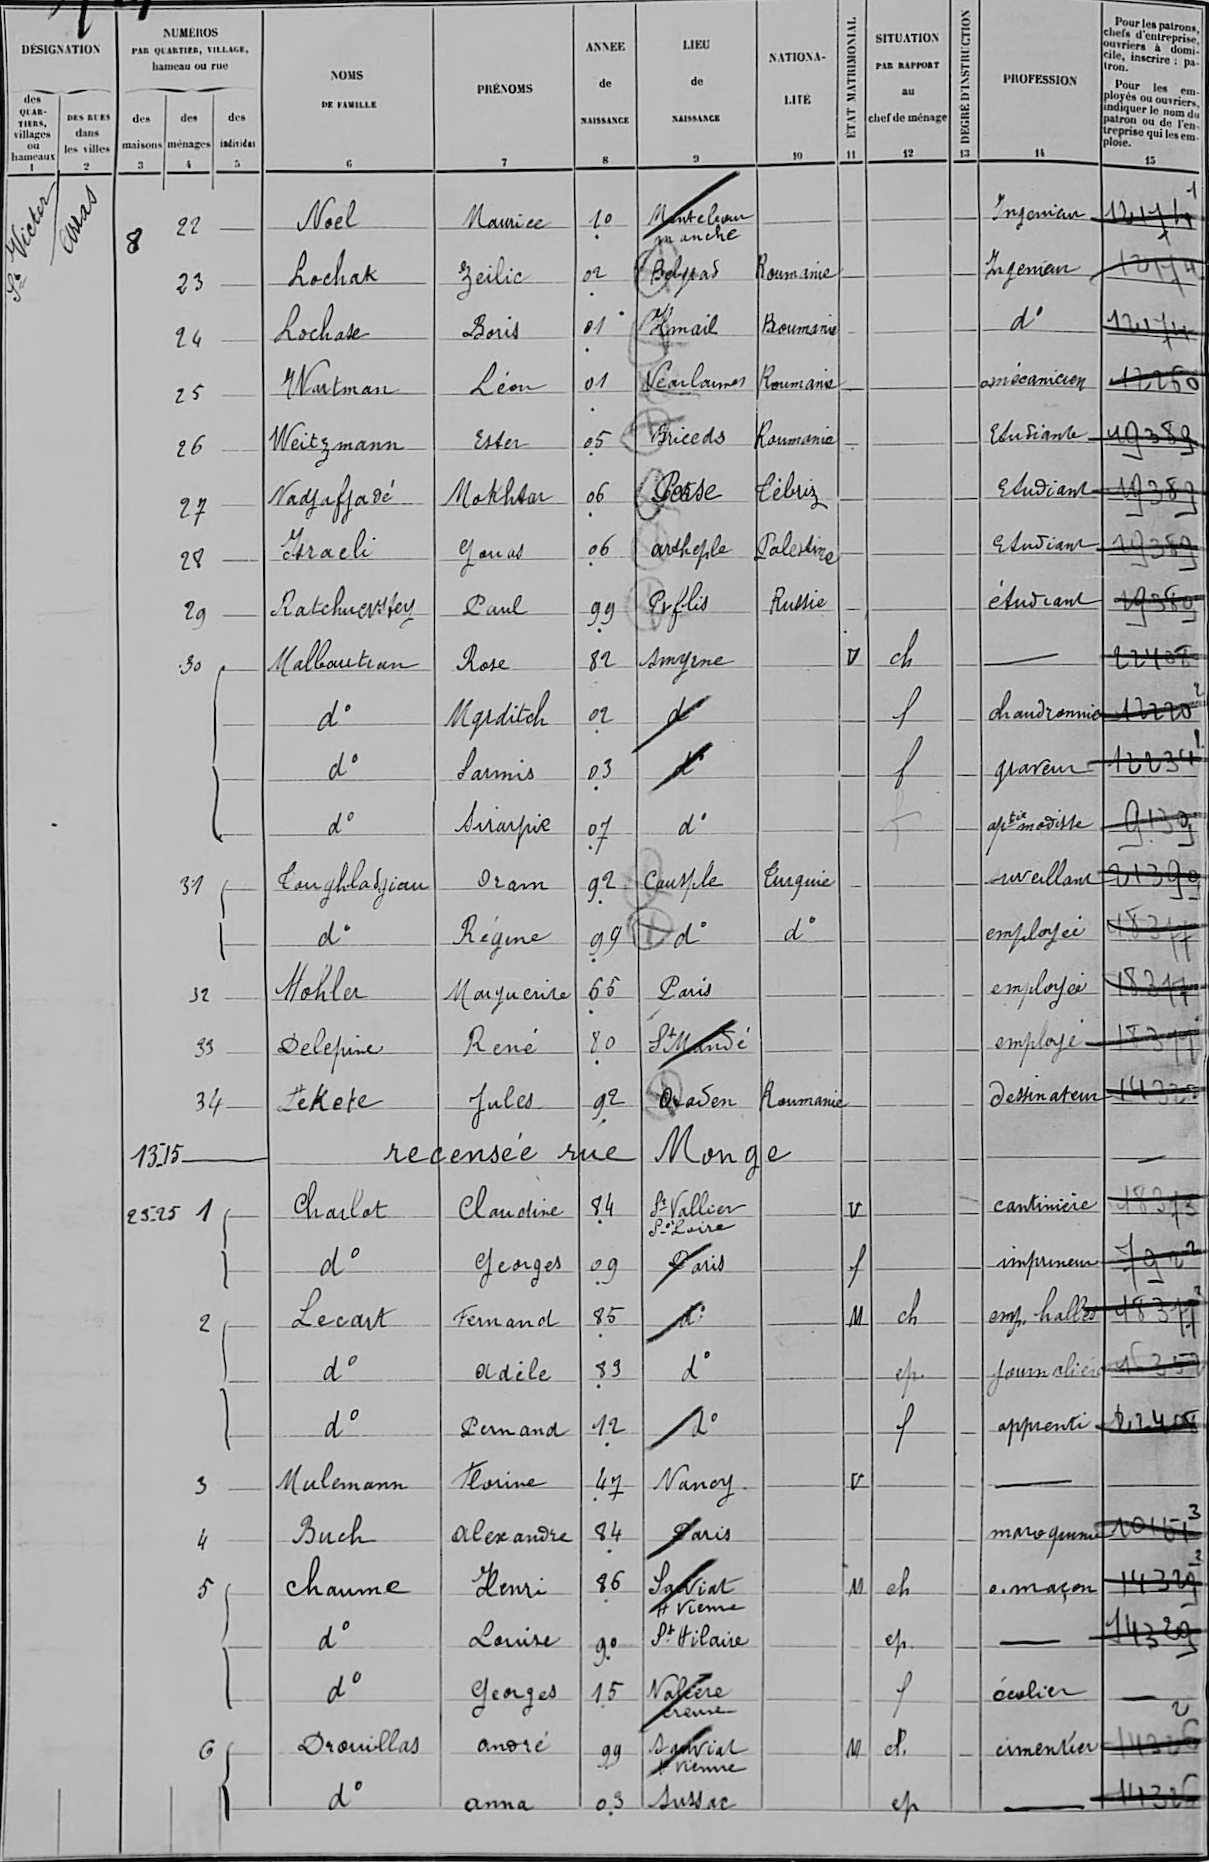Noël/Maurice/10/Monteleour !Manche/¤/¤/¤/¤/Ingénieur/12174
LochaK/Zeilie/02/Belgrad/Roumanie/¤/¤/¤/Ingenieur/12174
Lochase/Boris/01/Kmail/Roumanie/¤/¤/¤/d°/12174
Wutman/Léon/01/Verlaimes/Roumanie/¤/¤/¤/o mécanicien/12260
Weitzmann/Ester/05/Briceds/Roumanie/¤/¤/¤/Etudiante/19389
Nadjafjadé/Makhtar/06/Peae/Tébriz/¤/¤/¤/Etudiant/19389
Israeli/Gonas/06/artheple/Palestine/¤/¤/¤/Etudiant/19389
Ratchuerstey/Paul/99/Pvflis/Russie/¤/¤/¤/étudiant/19389
Malbautran/Rose/82/Smyrne/¤/V/ch/¤/¤/22408
d°/Mgrditch/02/d°/¤/¤/f/¤/chaudronnier/12220
d°/Sarmis/03/d°/¤/¤/f/¤/graveur/12234
d°/Sirarpie/07/d°/¤/¤/f/¤/aptie modiste/9139
Toughladgian/Oram/92/Caspile/Turquie/¤/¤/¤/surveillant/21399
d°/Régine/99/d°/d°/¤/¤/¤/employée/18377
Hohler/Marguerite/66/Paris/¤/¤/¤/¤/employée/18377
Delepine/René/80/St Mandé/¤/¤/¤/¤/employé/18399
Lekete/Jules/2/Ouaden/Roumanie/¤/¤/¤/dessinateur/14328
recensée rue Monge
Charlot/Claudine/84/St Vallier !S & Loire/¤/v/¤/¤/cantinière/18373
d°/Georges/09/Paris/¤/f/¤/¤/imprimeur/792
Lecart/Fernand/85/d°/¤/M/ch/¤/emp halles/18377
d°/Adèle/83/d0/¤/¤/ep /¤/journalière/16352
d°/Pernand/12/d°/¤/¤/f/¤/apprenti/22408
Mulemann/Florine/47/Nancy/¤/v/¤/¤/¤/¤
Buch/Alexandre/84/Paris/¤/¤/¤/¤/maroquinerie/10151
Chaume/Henri/86/Saviat !Hte Vienne/¤/M/ch/¤/o maçon/14329
d°/Louise/90/St Hilaire/¤/¤/ep /¤/¤/14329
d°/Georges/15/Naliere !Creuse/¤/¤/f/¤/écolier/¤
Drouillas/André/99/Sauviat !Hte Vienne/¤/M/ch /¤/cimentier/14326
d°/anna/03/Sussac/¤/¤/ep/¤/¤/14326
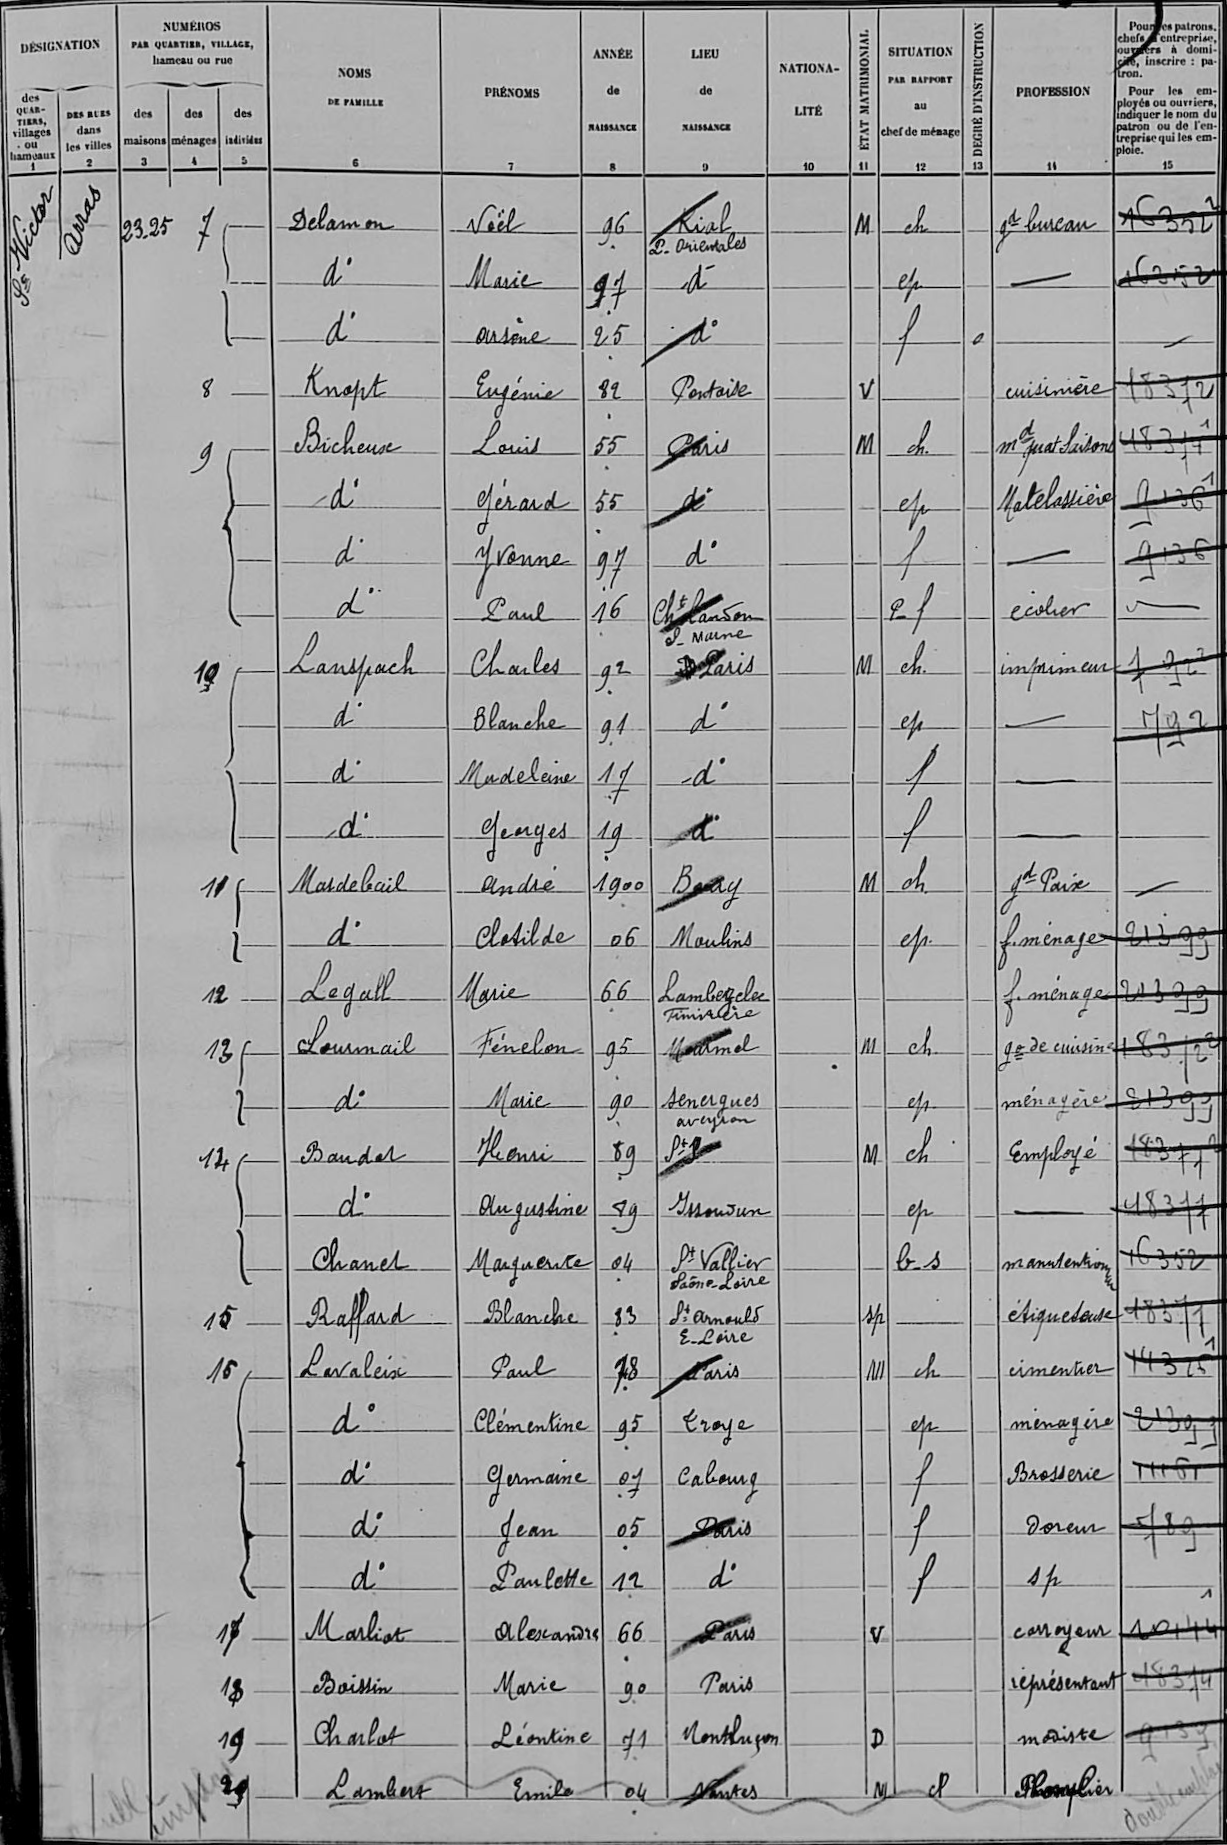Delamon/Noël/96/Rial !P Orientales/¤/M/ch/¤/gd bureau/16352
d°/Marie/97/d°/¤/¤/ep/¤/¤/16352
d°/arsène/25/d°/¤/¤/f/o/¤/¤
Knopt/Eugénie/82/Pontoise/¤/v/¤/¤/cuisinière/18372
Bicheux/Louis/55/Paris/¤/M/ch /¤/md quat Saisons/18374
d°/Gérard/55/d°/¤/¤/ep/¤/Matelassière/9136
d°/Yvonne/97/d°/¤/¤/f/¤/¤/9136
d°/Paul/16/Cht Landon ! S Marne/¤/¤/P f/¤/écolier/¤
Lanspach/Charles/92/Paris/¤/M/ch /¤/imprimeur/792
d°/Blanche/91/d°/¤/¤/ep/¤/¤/792
d°/Madeleine/17/d°/¤/¤/f/¤/¤/¤
d°/Georges/19/d°/¤/¤/f/¤/¤/¤
Masdebail/andré/1900/Bourg/¤/M/ch /¤/gd paix/¤
d°/Clotilde/06/Moulins/¤/¤/ep/¤/f Ménage/21399
Legall/Marie/66/Lambezelec !Finistère/¤/¤/¤/¤/f ménage/21399
Lourmail/Fénelon/95/Mourmel/¤/M/ch/¤/go de cuisine/18372
d°/Marie/90/Senergues ! AVeyron/¤/¤/ep/¤/ménagère/81399
Baudet/Henri/89/St S/¤/M/ch/¤/Employé/18377
d°/Augustine/89/Issoudun/¤/¤/ep/¤/¤/18377
Chanet/Marguerite/04/St Vallier ! Saône Loire/¤/¤/b -s/¤/manutentionnaire/16352
Raffard/Blanche/83/St Arnould !E Loire/¤/sp/¤/¤/étiqueteuse/18377
Lavaleix/Paul/78/Paris/¤/M/ch/¤/cimentier/14326
d°/Clémentine/95/Troye/¤/¤/ep/¤/ménagère/21399
d°/Germaine/07/Cabourg/¤/¤/f/¤/Brosseire/11161
d°/Jean/05/Paris/¤/¤/f/¤/doreur/789
d°/Paulette/12/d°/¤/¤/f/¤/sp/¤
Marliot/Alexandre/66/Paris/¤/v/¤/¤/corroyeur/10144
Boissin/Marie/90/Paris/¤/¤/¤/¤/représentant/18374
Charlot/Léontine/71/Montluçon/¤/D/¤/¤/modiste/9139
Lambert/Emile/04/Nantes/¤/M/ch/¤/Plombier/¤